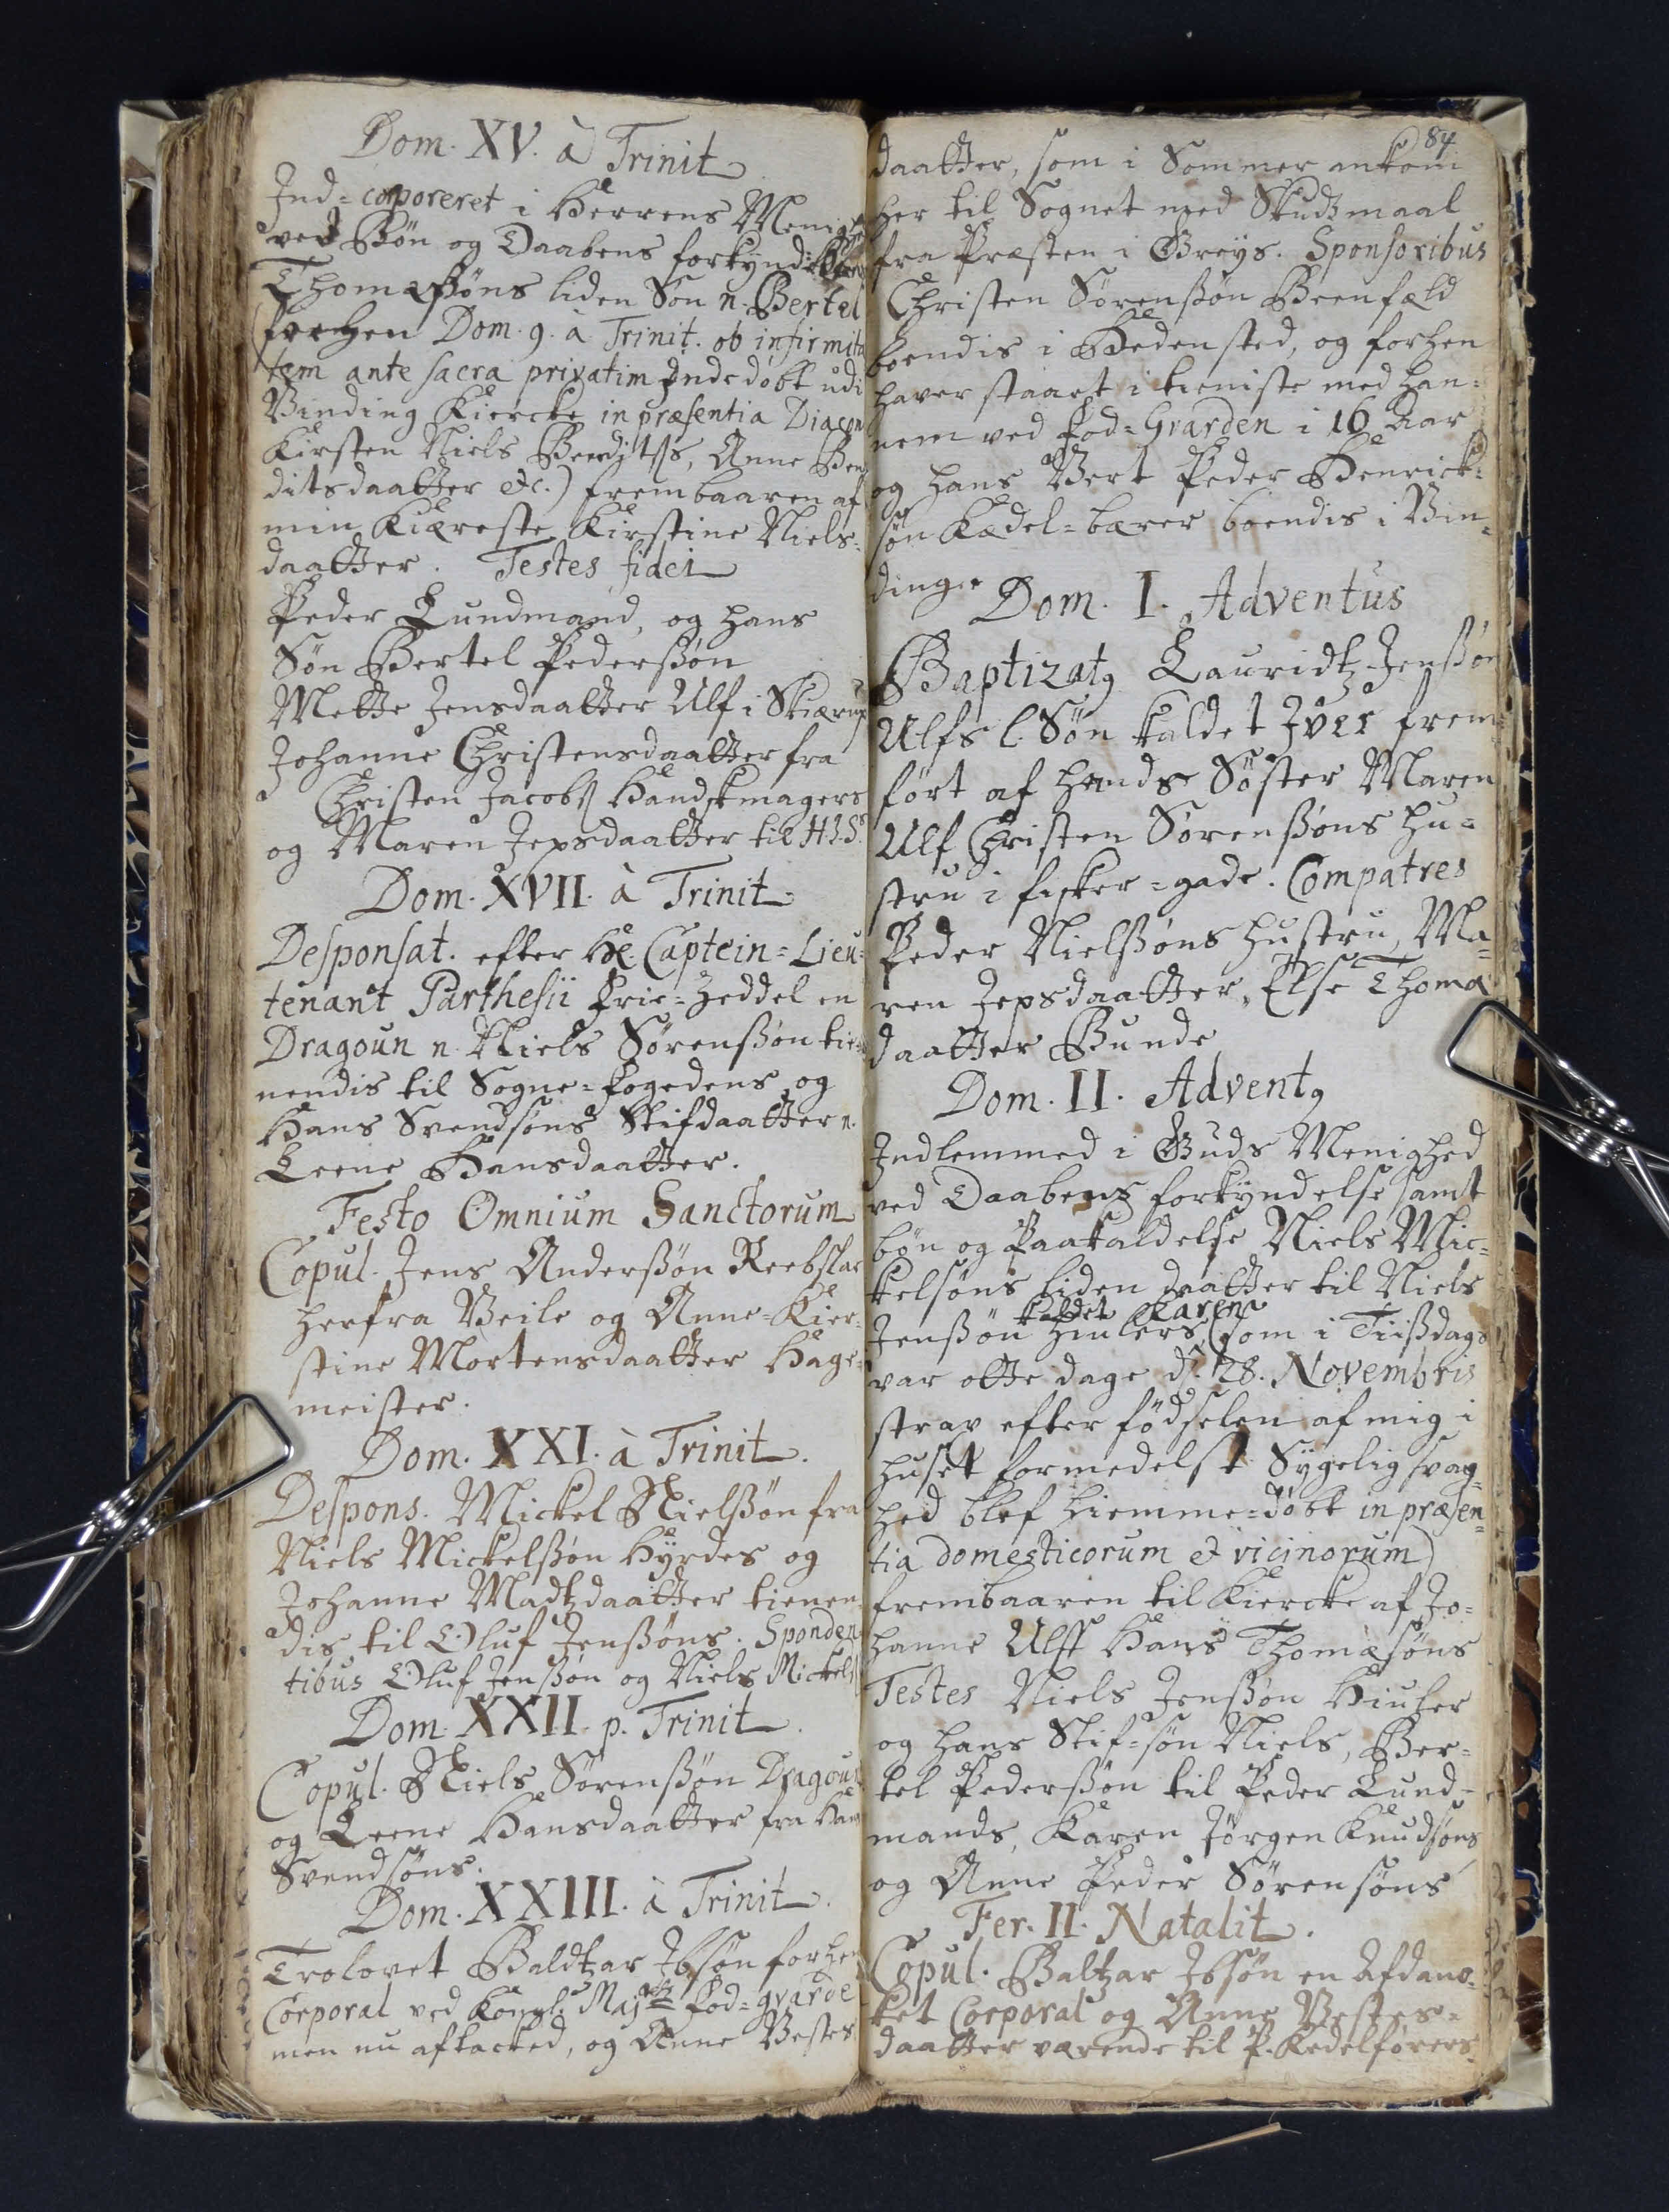Dom. XV. a Trinit
Ind = copoceret i Herrens Menighe 
ved Bøn og Daabens forkynde##
Thomæssøns liden Søn n. Bertel
(forhen Dom. 9. a Trinit. ob infirmita
tem ante sacra privatim Inde døbt udi
Vinding Kiercke in præsentia Diacon 
Kirsten Niels Bendtiss, Anne Ben
ditsdaatter etc.) frembaaren af
min Kiæreste Kirstine Niels¬
daatter. Testes fidei
Peder Lundmand, og hans
Søn Bertel Pedersøn
Mette Jensdaatter Ulf i Skiærup
Johanne Christensdaatter fra
Christen Jacobs Hanskemagers
og Maren Jepsdaatter til H.J.Ss.
Dom. XVII. a Trinit
Desponsat. efter Hl Captein=Lieu¬
tenant Parthesii Frie=Zeddel en
Dragoun n. Niels Sørenssøn tie¬
nendis til Sogne=fogedens og
Hans Svendsøns Stifdaatter n.
Leene Hansdaatter.
Festo Omnium Sanctorum
Copul. Jens Anderssøn Reebslar
herfra Veile og Anne= Kier¬
stine Mortensdaatter Hage¬
meister.
Dom. XXI. a Trinit.
Despons. Mickel Nielssøn fra
Niels Mickelssøn Hyrdes og
Johanne Madtzdaatter tienen¬
dis til Oluf Jenssøns. Sponden¬
tibus Oluf Jenssøn og Niels Mickels
Dom. XXII. p. Trinit
Copul. Niels Sørenssøn Dragoun
og Leene Hansdaatter fra Han 
Svendsøns.
Dom. XXIII. a Trinit
Trolovet Baldtzar Ibsøn forhen
Corporal ved Kongl. Majets Fod=qvarde
men nu aftacked, og Anne Vestes
84.
daatter, som i Sommer ankom
her til Sognet med Skudsmaal
fra Præsten i Greys. Sponsoribus
Christen Sørenssøn Beenfæld
boendis i Hedensted, og forhen
haver staaet i tieniste med han¬
nem ved Fod=Qvarden i 16 Aar
og hans Vert Peder Henrick¬
søn Kædel=bærer boendis i Vin¬
ding.
Do. I. Adventus
Baptizatq Lauridtz Jenssøn
Ulfs l. Søn kaldet Iver, frem
ført af hands Søster Maren
Ulf Christen Sørenssøns hu¬
stru i fisker=gade. Compatres
Peder Nielssøns hustru, Ma¬
ren Jepsdaatter, Else Thoma 
daatter Bunde.
Dom. II. Adventq
Indlemmed i Guds Menighed
ved Daabens forkyndelse samt 
bøn og Paakaldelse Niels Mic¬
kelsøns liden daatter til Niels
kaldet Karen
Jenssøn Hiulers, (som i Tiissdags
var otte dage d. 28. Novembris
strax efter fødselen af mig i
huset formedelst Sygelig svag¬
hed blef hiemme=døbt in præsen¬
tia domesticorum et vicinorum)
frembaaren til Kiercke af Jo¬
hanne Ulff Hans Thomæsøns
Testes Niels Jenssøn Hiuler
og hans Stif=søn Niels, Ber¬
tel Pederssøn til Peder Lund¬
mands, Karen Jørgen Knudsøns
og Anne Peder Sørensøns
Fer. II. Natalit
Copul. Baltzar Ibsøn en Afdanc¬
ket Corporal og Anne Vestes¬
daatter værendis til P. Kedelførers.